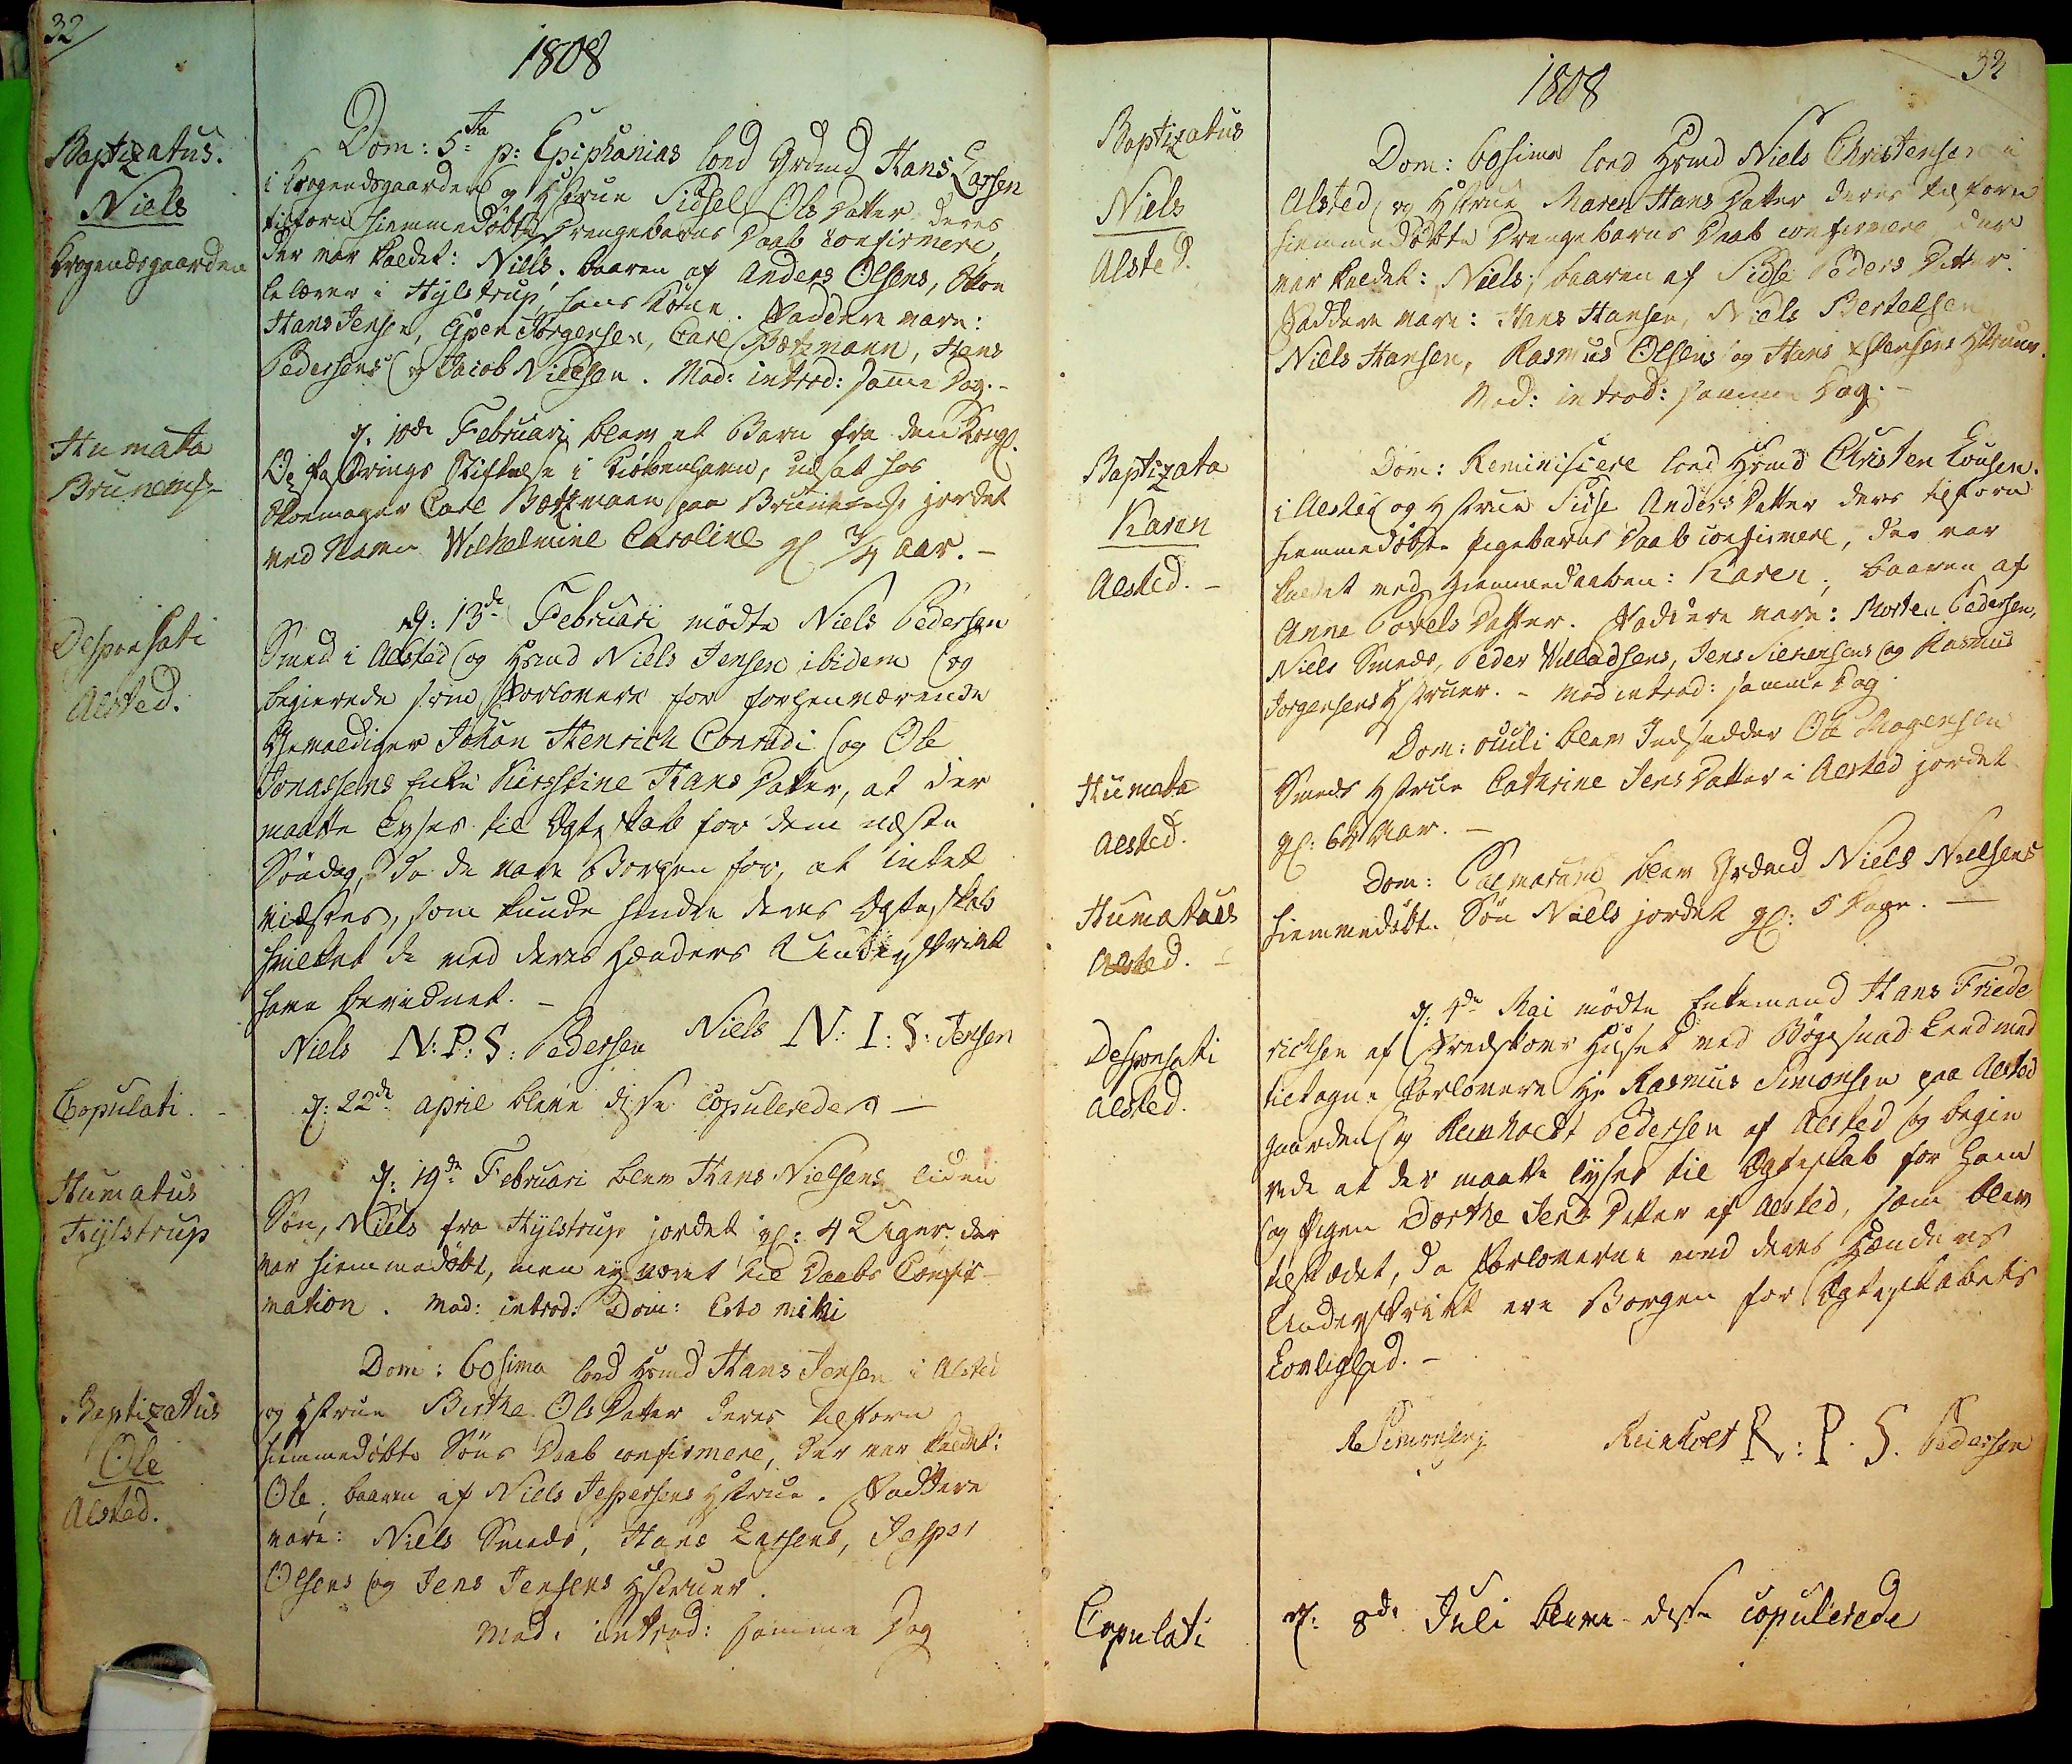32
Baptizatus
Niels
Krogendegaarden
Humata
Brunem##
Desponsati
Alsted.
Copulati
Humatus
Hylstrup
Baptizayus
Ole
Alsted.
1808
Dom: 5ta p: Epiphanias loed Grdmd Hans Larsen
i Krogendegaarden og Hstrue Sidsel Ols Datter deres
tilforn hiemmedøbte Drengebarns Daab confirmere,
der var kaldet: Niels. baaren af Anders Olsens, Skoe
lelærer i Hylstrup hans Kone. Faddere vare;
Hans Jensen, Espen Jørgensen, Carl Bætzmann, Hans
Pedersens og Jacob Nielsen. Mod: introd: samme Dag.
d: 10de Februari blev et Barn fra den Kongl.
Opfostrings Stiftelse i Kiøbenhavn, udsat hos
Skoemager, Karl Bætzmann paa Brunm## jordet
ved Navn Wilhelmine Caroline gl ¾ Aar.
d: 13de Februari mødte Niels Pedersen
Smed i Alsted og Hsmd Niels Jensen ibidem og
begierede, som Forlovere for forhenværende
Gevaldiger Johan Henrich Conradi og Ole
Jonassens Enke Kirstine Hans Datter, at der
maatte lyses til Ægteskab for dem næste
Søndag, da de være Boesen for, at intet
vidstes, som kunde hindre deres Ægteskab
hvilket de med deres Hænders Underskrivt
have bevidnet.
Niels N: P: S: Pedersen Niels N: I: S: Jensen
d: 22de April bleve disse copulerede
d: 19de Februari blev Hans Nielsens liden
Søn, Niels fra Hylstrup jordet gl: 4 Uger. der
var hiemmedøbt, men ey været til Daab Confir¬
mation. Mod: introd: Dom: Esto mihi
Dom: 60sima loed Hsmd Hans Jensen i Alsted
og Hstrue Birthe Ols Datter deres tilforn
hiemmedøbte Søns Daab confirmere, der var kaldet:
Ole; baaren af Niels Jespersens Hstrue. Faddere
vare: Niels Smeds, Hans Lassens, Jesper
Olsens og Jens Jensens Hstruer.
Mod: introd: samme Dag
Baptizatus
Niels
Alsted.
Baptizata
Karen
Alsted.
Humata
Alsted
Humatus
Alsted
Desponsati
Alsted.
Copulati
33
1808
Dom: 60sima loed Hsmd Niels Christensen i
Alsted og Hstrue Maren Hans Datter deres tilforn
hiemmedøbte Drengebarns Daab confirmere der
var kaldet: Niels; baaren af Sidse Peders Datter.
Faddere vare: Hans Hansen, Niels Bertelsen
Niels Hansen, Rasmus Olsens og Hans Xstensens Hstrue.
Mod: introd: samme Dag.
Dom: Reminiscere loed Hsmd Christen Lousen.
i Alsted og Hstrue Sidse Anders Datter deres tilforn
hiemmedøbte Pigebarns Daab confirmere, der var
kaldet ved Hiemmedaaben: Karen; baaren af
Anne Povels Datter. Faddere vare: Morten Pedersen,
Niels Smeds, Peder Villadsens, Jens Silkensens og Rasmus
Jørgensens Hstruer. - Mod introd: samme Dag
Dom: oculi blev Indsidder Ole Mogensen
Smeds Hstrue Cathrine Jens Datter i Alsted jordet
gl: 64 Aar.
Dom: Palmarum blev Grdmd Niels Nielsens
hiemmedøbte Søn Niels jordet gl: 5 Dage.
d: 4de Mai mødte Enkemand Hans Friede
richsen af Fredskovs Huset ved Bøges##d L##d med
tiltagne Forlovere Hr Rasmus Simonsen paa Alsted
gaarden og Reinholdt Pedersen af Alsted og begie
rede at der maatte lyses til Ægteskab for ham
og Pigen Dorthe Jens Datter af Alsted, som blev
tilstædet, da Forloverne ved deres Hænders
Underskrivt ere Borgen for Ægteskabets
Lovlighed.
R Simonsen Reinholt R: P: S: Pedersen
d:. 8de Juli bleve disse copulerede
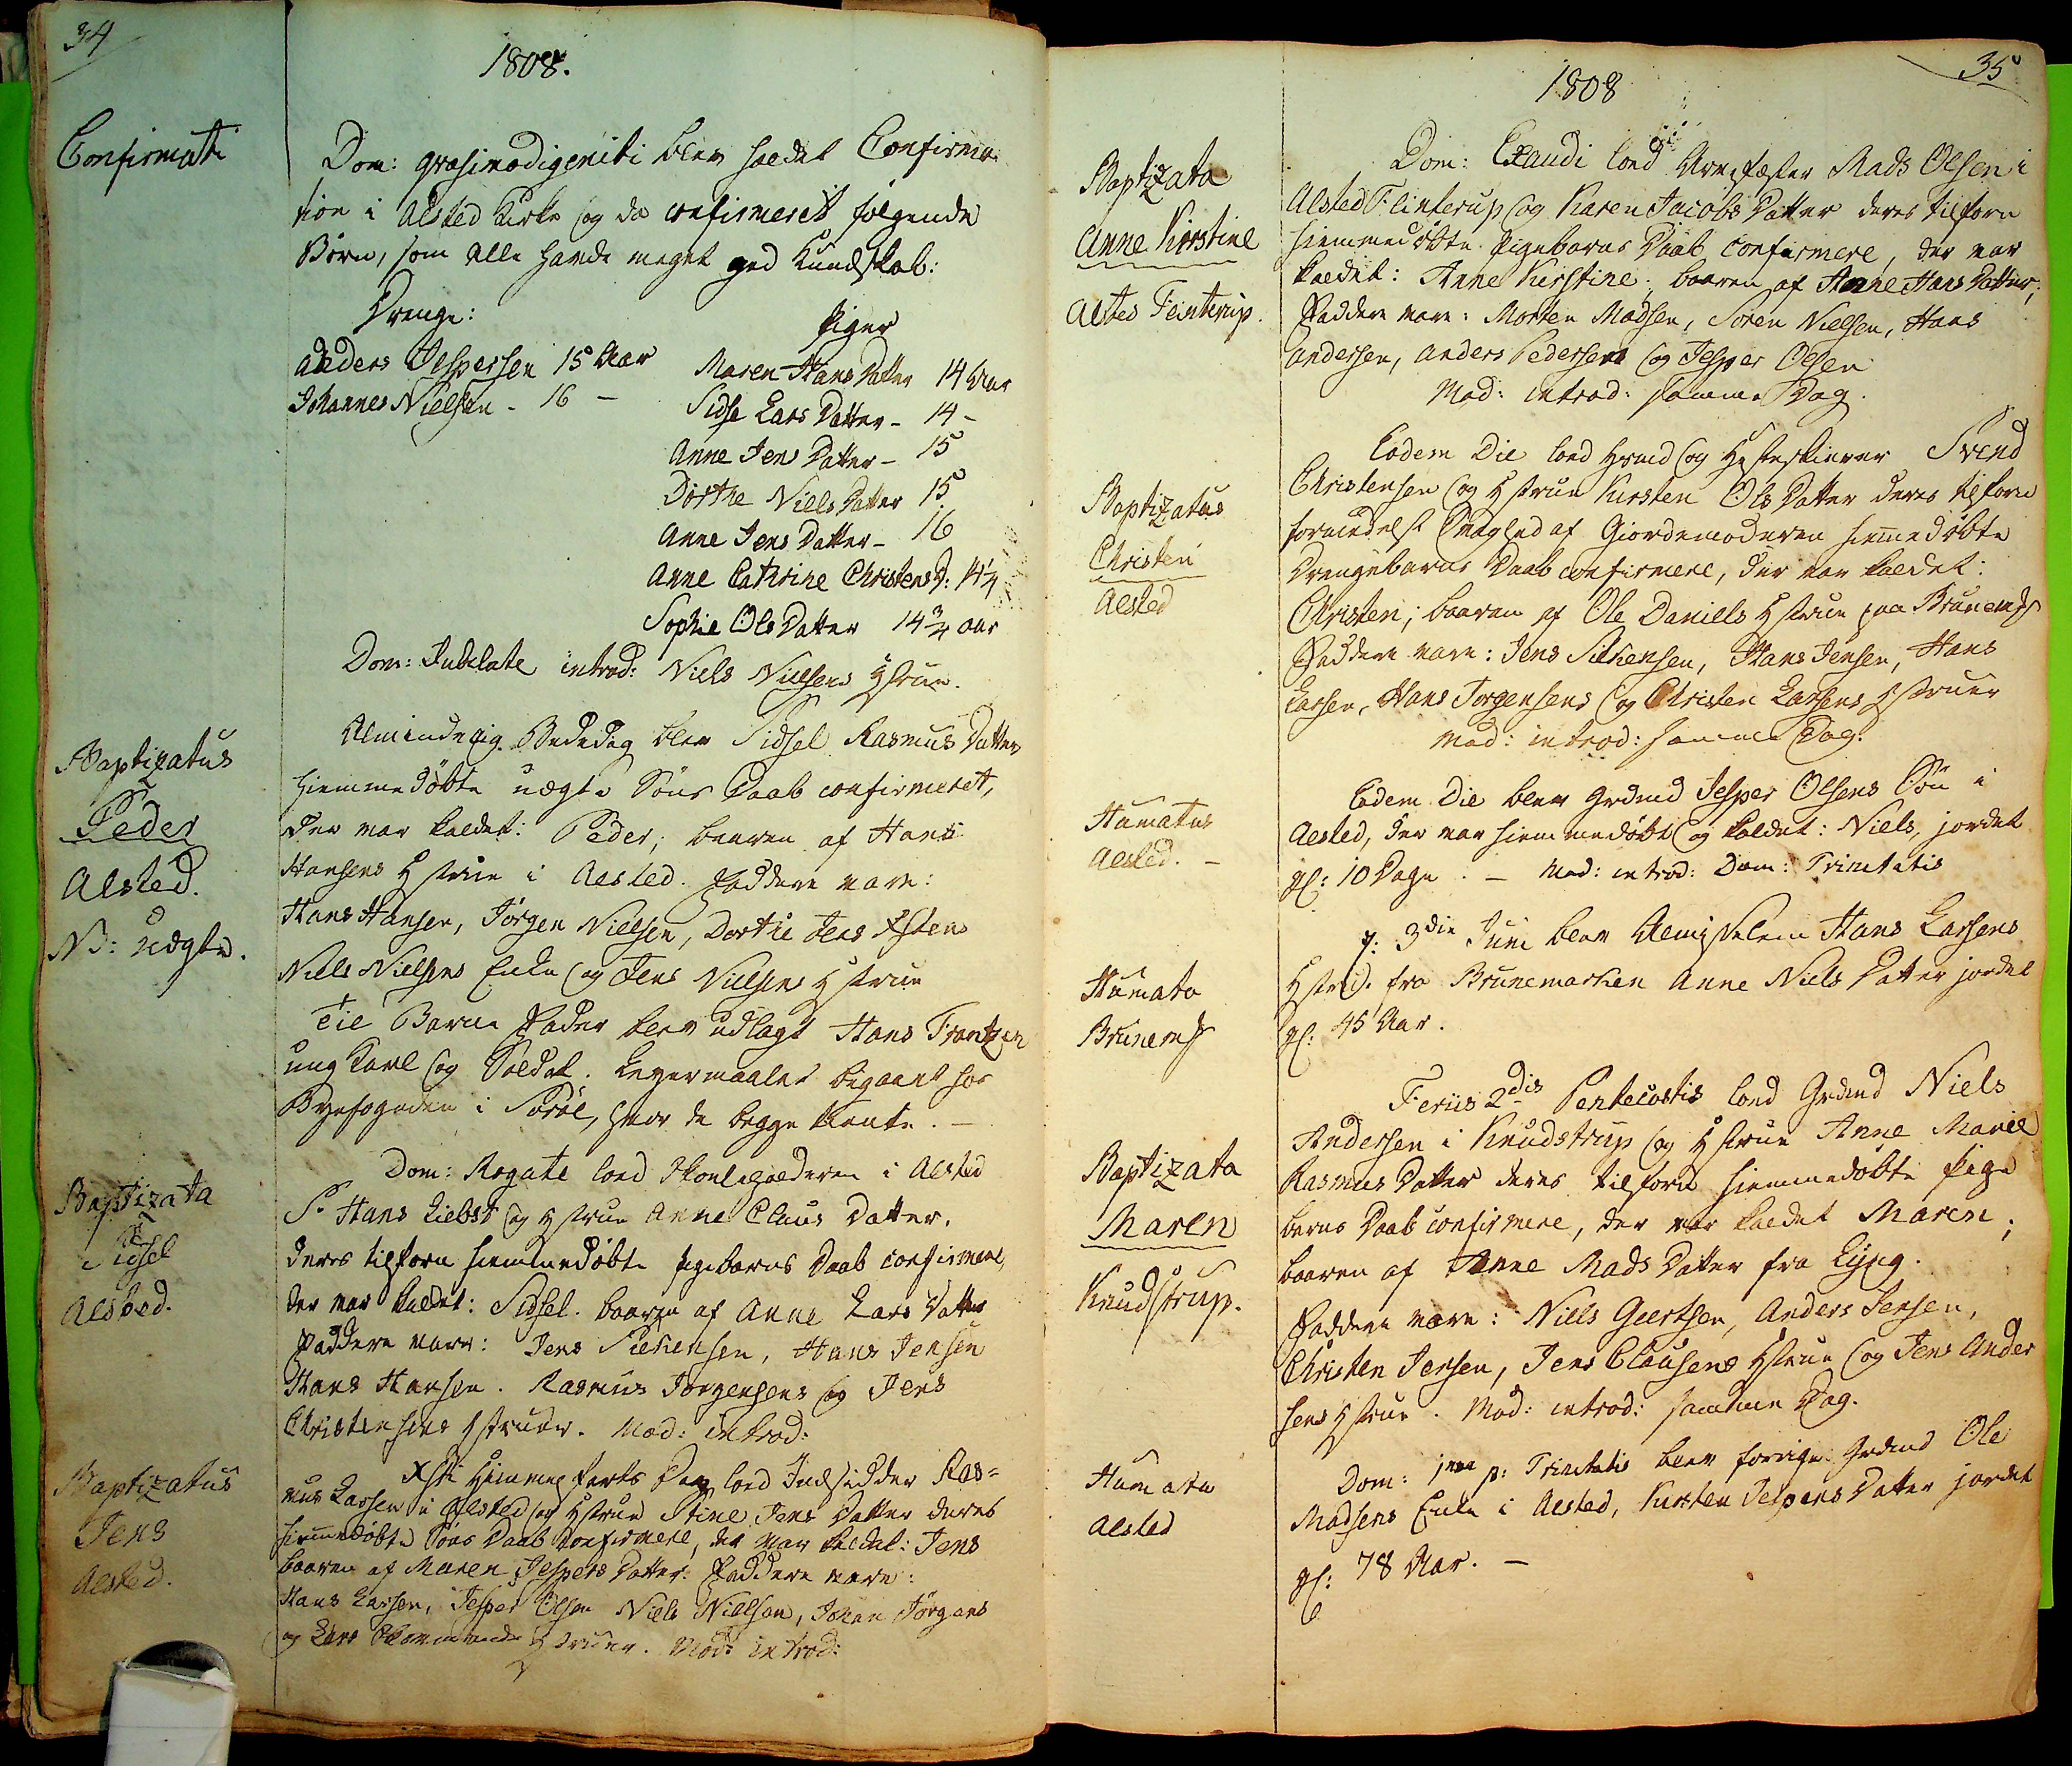34
Confirmati
Baptizatus
Peder
Alsted.
NB: uægte
Baptizata
Sidsel
Alsted:
Baptizatus
Jens
Alsted.
1808.
Dom: Qvasimodigeniti blev holdet Confirma
tion i Alsted Kirke og da confirmeret følgende
Børn, som alle havde meget god Kundskab:
Drenge:
Anders Jespersen 15 Aar
Johannes Nielsen - 16 -
Pige
Maren Hans Datter 14 Aar
Sidse Lars Datter - 14 -
Anne Jens Datter - 15
Dorthe Niels Datter 15
Anne Jens Datter - 16
Anne Cathrine Christens D: 14¼
Sophie Ols Datter 14 ¾ Aar
Dom: Jubilate introd: Niels Nielsens Hstrue.
Almindelig Bededag blev Sidsel Rasmus Datters
hiemmedøbte uægte Søns Daab confirmeret,
der var kaldet: Peder; baaren af Hans
Hansens Hstrue i Alsted. Faddere vare:
Hans Hansen, Jørgen Nielsen, Dorthe Jens Xstens
Niels Nielsens Enke og Jens Nielsens Hstrue
Til Barne Fader blev udlagt Hans Frantzen
ungkarl og Soldat. Leyermaallet begaaet hos
Byefogeden i Sorøe, hvor de begge tiente.
Dom: Rogate loed Skoeleholderen i Alsted
S. Hans Liebst og Hustrue Anne Claus Datter.
deres tilforn hiemmedøbte Pigebarns Daab confirmere
der var kaldet: Sidsel. baaren af Anne Lars Datter
Faddere vare: Jens Silkensen, Hans Jensen
Hans Hansen. Rasmus Jørgensens og Jens
Christensens Hstruer. Mad: introd:
Xsti Hiemelfarts Dag loed Indsidder Ras¬
mus Lassen i Alsted og Hstrue Stine Jens Datter deres
hiemmedøbte Søns Daab confirmene, der var kaldet: Jens
baaren af Maren Jespers Datter: Faddere vare:
Hans Lassen, Jesper Olsen, Niels Nielsen, Johan Jørgens
og Lars Skovmands Hstruer. Mod: introd:
Baptizata
Anne Kirstine
Alsted Flinterup
Baptizatus
Christen
Alsted
Humatus
Alsted
Humata
Brunem##
Baptizata
Maren
Knudstrup.
Humata
Alsled
35
1808
Dom: Exaudi loed Arvefæster Mads Olsen i
Alsted Flinrerup og Karen Jacobs Datter deres tilforn
hiemmedøbte Pigebarns Daab Confirmere, der var
kaldet: Anne Kirstine. baaren af Anne Hans Datter
Faddere vare: Morten Madsen, Søren Nielsen, Hans
Andersen, Anders Pedersen og Jesper Olsen
Mod: introd: samme Dag.
Eodem Die loed Hsmd og Hesteskierer Svend
Christensen og Hstrue Kirsten Ols Datter deres tilforn
formedelst svaghed af Giordemoderen hiemmedøbte
Drengebarns Daab confirmere, der var kaldet:
Christen; baaren af Ole Daniels Hstrue paa Brunem##
Faddere vare: Jens Silkensen, Hans Jensen, Hans
Larsen, Hans Jørgensens og Christen Larsens Hstruer
Mod: introd: samme Dag.
Eodem Die blev Grdmd Jesper Olsens Søn i
Alsted, der var hiemmedøbt og kaldet: Niels, jordet
gl: 10 Dage. - Mod: introd: Dom: Trinitatis
d: 3die Juni blev Almisselen Hans Larsens
Hstrue fra Brunemarken Anne Niels Datter jordet
gl: 45 Aar.
Feriis 2dis Pentecostis loed Grdmd Niels
Anderssen i Knudstrup og Hstrue Anne Marie
Rasmus Datter deres tilforn hiemmedøbte Pige
barns Daab confirmere, der var kaldet Maren;
baaren af Anne Mads Datter fra Lyng.
Faddere vare: Niels Geertsen, Anders Jensen,
Christen Jensen, Jens Clausens Hstrue og Jens Ander
sens Hstrue. Mod: introd: samme Dag.
Dom: 1ma p: Trinitatis blev forrigen Grdmd Ole
Madsens Enke i Alsted, Kirsten Jespers Datter jordet
gl: 78 Aar.